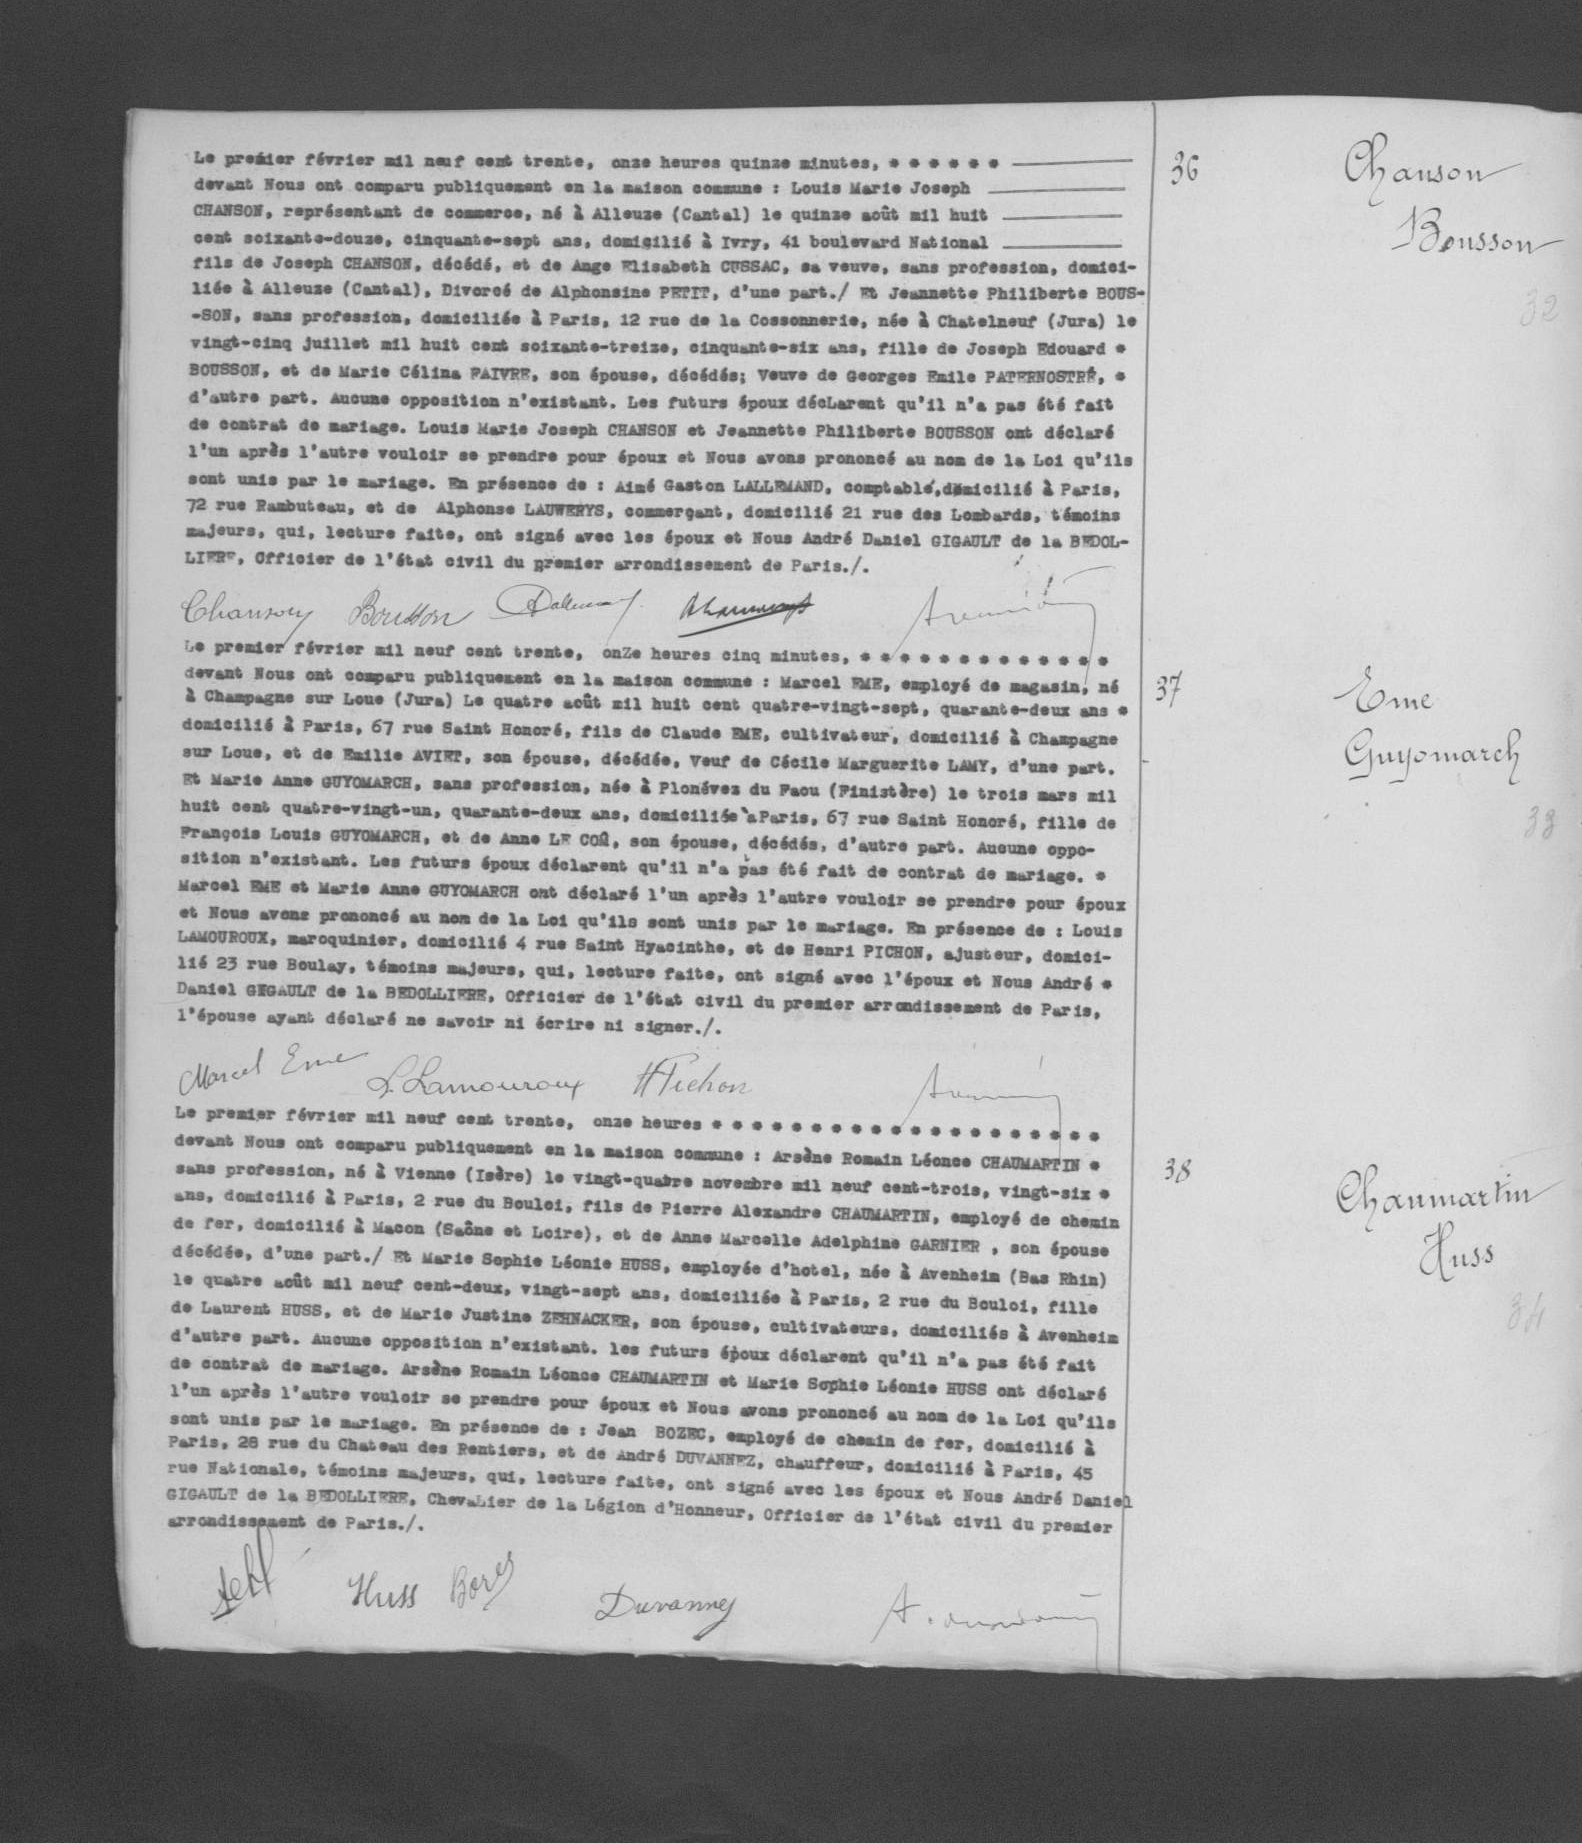Le premier février mil neuf cent trente, onze heures quinze minutes, **** -
devant Nous ont comparu publiquement en la maison commune: Louis Marie Joseph -
CHANSON, représentant de commerce, né à Alleuze (Cantal) le quinze août mil huit -
cent soixante-douze, cinquante-sept ans, domicilié à Ivry, 41 boulevard National -
fils de Joseph CHANSON, décédé, et de Ange Elisabeth CUSSAC, sa veuve, sans profession, domici-
lié à Alleuze (Cantal), Divorcé de Alphonsine PETIT, d'une part ./ Et Jeannette Philiberte BOUS-
-SON, sans profession, domiciliée à Paris, 12 rue de la Cossonnerie, née à Chatelneuf (Jura) le
vingt-cinq juillet mil huit cent soixante-treize, cinquante-six ans, fille de Joseph Edouard *
BOUSSON, et de Marie Céline FAIVRE, son épouse, décédés; Veuve de Georges Emile PATERNOSTRE, *
d'autre part. Aucune opposition n'existant. Les futurs époux déclarent qu'il n'a pas été fait
de contrat de mariage. Louis Marie Joseph CHANSON et Jeannette Philiberte BOUSSON ont déclaré
l'un après l'autre vouloir se prendre pour époux et Nous avons prononcé au nom de la Loi qu'ils
sont unis par le mariage. En présence de: Aimé Gaston LALLEMAND, comptable, domicilié à Paris,
72 rue Rambuteau, et de Alphonse LAUWERYS, commerçant, domicilié 21 rue des Lombards, témoins
majeurs, qui, lecture faite, ont signé avec les époux et Nous André Daniel GIGAULT de la BEDOL-
LIERE, Officier de l'état civil du premier arrondissement de Paris ./.
Le premier février mil neuf cent trente, onze heures cinq minutes, ****
devant Nous ont comparu publiquement en la maison commune: Marcel EME, employé de magasin, né
à Champagne sur Loue (Jura) Le quâtre août mil huit cent quatre-vingt-sept, quarante-deux ans *
domicilié à Paris, 67 rue Saint Honoré, fils de Claude EME, cultivateur, domicilié à Champagne
sur Loue, et de Emilie AVIRT, son épouse, décédée. Veuf de Cécile Marguerite LAMY, d'une part.
Et Marie Anne GUYOMARCH, sans profession, née à Polneves du Faou (Finistère) le trois mars mil
huit cent quatre-vingt-un, quarante-deux ans, domiciliée à Paris, 67 rue Saint Honoré, fille de
François Louis GUYOMARCH, et de Anne LE COM, son épouse, décédés, d'autre part. Aucune oppo-
sition n'existant. Les futurs époux déclarent qu'il n'a pas été fait de contrat de mariage. *
Marcel EME et Marie Anne GUYOMARCH ont déclaré l'un après l'autre vouloir se prendre pour époux
et Nous avons prononcé au nom de la Loi qu'ils sont unis par le mariage. En présence de: Louis
LAMOUROUX, maroquinier, domicilié 4 rue Saint Hyacinthe, et de Henri PICHON, ajusteur, domici-
lié 23 rue Boulay, témoins majeurs, qui, lecture faite, ont signé avec l'époux et Nous André *
Daniel GIGAULT de la BEDOLLIERE, Officier de l'état civil du premier arrondissement de Paris,
l'épouse ayant déclaré ne savoir ni écrire ni signer ./.
Le premier février mil neuf cent trente, onze heures ****
devant Nous ont comparu publiquement en la maison commune: Arsène Romain Léonce CHAUMARTIN *
sans profession, né à Vienne (Isère) le vingt-quatre novembre mil neuf cent-trois, vingt-six *
ans, domicilié à Paris, 2 rue du Bouloi, fils de Pierre Alexandre CHAUMARTIN, employé de chemin
de fer, domicilié à Macon (Saône-et-Loire), et de Anne Marcelle Adelphine GARNIER, son épouse
décédée, d'une part ./ Et Marie Sophie Léonie HUSS, employée d'hotel, née à Avenheim (Bas Rhin)
le quatre août mil neuf cent-deux, vingt-sept ans, domiciliée à Paris, 2 rue du Bouloi, fille
de Laurent HUSS, et de Marie Justine ZEHNACKER, son épouse, cultivateurs, domiciliés à Avenheim
d'autre part. Aucune opposition n'existant. les futurs époux déclarent qu'il n'a pas été fait
de contrat de mariage. Arsène Romain Léonce CHAUMARTIN et Marie Sophie Léonie HUSS ont déclaré
l'un après l'autre vouloir se prendre pour époux et Nous avons prononcé au nom de la Loi qu'ils
sont unis par le mariage. En présence de: Jean Bozec, employé de chemin de fer, domicilié à
Paris, 28 rue du Chateau des Rentiers, et de André DUVANNEZ, chauffeur, domicilié à Paris, 45
rue Nationale, témoins majeurs, qui, lecture faite, ont signé avec les époux et Nous André Daniel
GIGAULT de la BEDOLLIERE, Chevalier de la Légion d'Honneur, Officier de l'état civil du premier
arrondissement de Paris ./.
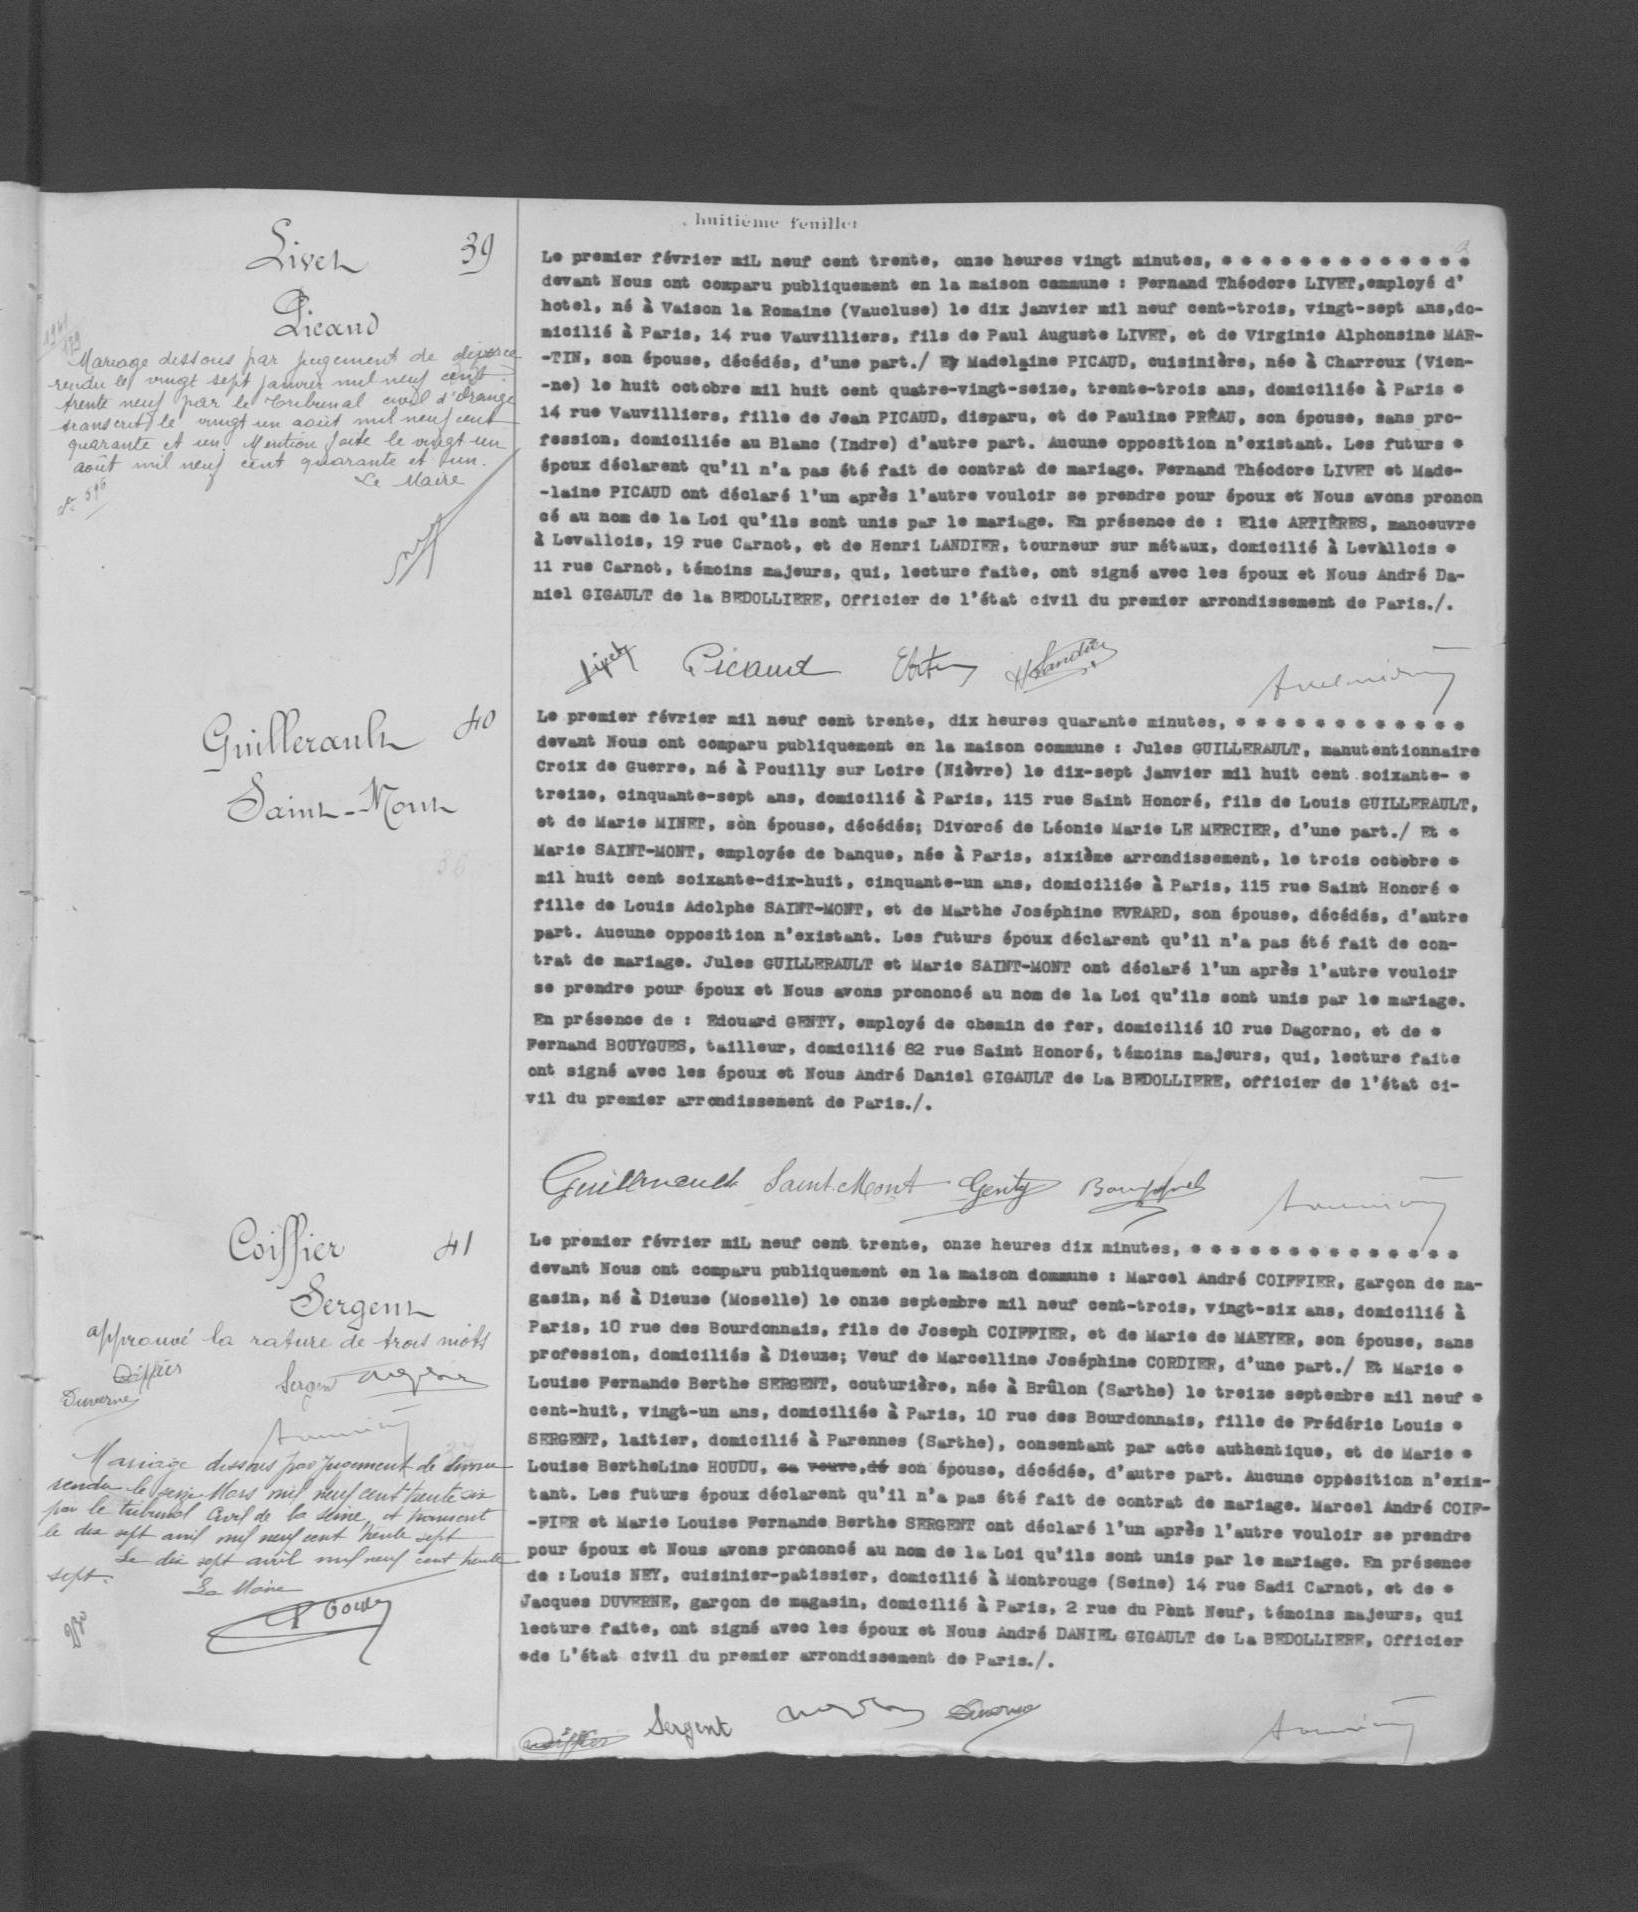Le premier février mil neuf cent trente, onze heures vingt minutes, ****
devant Nous ont comparu publiquement en la maison commune: Fernand Théodore LIVET, employé d'
hotel, né à Vaison la Romaine (Vaucluse) le dix janvier mil neuf cent-trois, vingt-sept ans, do-
micilié à Paris, 14 rue Vauvilliers, fils de Paul Auguste LIVET, et de Virginie Alphonsine MAR-
-TIN, son épouse, décédés, d'une part ./ Et Madelaine PICAUD, cuisinière, née à Charroux (Vien-
-ne) le huit octobre mil huit cent quatre-vingt-seize, trente-trois ans, domiciliée à Paris *
14 rue Vauvilliers fille de Jean PICAUD, disparu, et de Pauline PREAU, son épouse, sans pro-
fession, domiciliée au Blanc (Indre) d'autre part. Aucune opposition n'existant. Les futurs *
époux déclarent qu'il n'a pas été fait de contrat de mariage. Fernand Théodore LIVET et Made-
-laine PICAUD ont déclaré l'un après l'autre vouloir se prendre pour époux et Nous avons pronon
cé au nom de la Loi qu'ils sont unis par le mariage. En présence de: Elie ARTIERES, manoeuvre
à Levallois, 19 rue Carnot, et de Henri LANDIER, tourneur sur métaux, domicilié à Levallois *
11 rue Carnot, témoins majeurs, qui, lecture faite, ont signé avec les époux et Nous André Da-
niel GIGAULT de la BEDOLLIERE, Officier de l'état civil du premier arrondissement de Paris ./.
Le premier février mil neuf cent trente, dix heures quarant minutes, ****
devant Nous ont comparu publiquement en la maison commune: Jules GUILERAULLT, manutentionire
Croix de Guerre, né à Pouilly sur Loire (Nièvre) le dix-sept janvier mil huit cent soixante-*
treize, cinquante-sept ans, domicilié à Paris, 115 rue Saint Honoré, fils de Louis GUILLERAULT,
et de Marie MINET, son épouse, décédés; Divorcé de Léonie Marie LE MERCIER, d'une part ./ Et *
Marie SAINT-MONT, employée de banque, née à Paris, sixième arrondissement, le trois octobre *
mil huit cent soixante-dix-huit, cinquante-un ans, domiciliée à Paris, 115 rue SAint Honoré *
fille de Louis Adolphe SAINT-MONT, et de Marthe Joséphine EVRARD, son épouse, décédés, d'autre
part. Aucune opposition n'existant. Le futurs époux déclarent qu'il n'a pas été fait de cont
rat de mariage. Jules GUILLERAULT et Marie SAINT-MONT ont déclaré l'un après l'autre vouloir
se prendre pour époux et Nous avons prononcé au nom de la Loi qu'ils sont unis par le mariage.
En présence de: Edouard GENTY, employé de chemin de fer, domicilié 10 rue DAGORNO, et de *
Fernand BOUYGUES, tailleur, domicilié 82 rue Saint Honoré, témoins majeurs, qui, lecture faite
ont signé avec les époux et Nous André Daniel GIGAULT de la BEDOLLIERE, officier de l'état ci-
vil du premier arrondissement de Paris ./.
Le premier février mil neuf cent trente, onze heures dix minutes, ****
devant Nous ont comparu publiquement en la maison commune: Marcel André COIFFIER, garçon de ma-
gasin, né à Dieuse (Moselle) le onze septembre mil neuf cent-trois, vingt-six ans, domicilié à
Paris, 10 rue des Bourdonnais, fils de Joesph COIFFIER, et de Marie de MAEYER, son épouse, sans
profession, domiciliée à Dieuse; Veuf de Marcelline Joséphine CORDIER, d'une part ./ Et Marie *
Louise Fernande Berthe SERGENT, couturière, née à Brûlon (Sarthe), le treize septembre mil neuf *
cent-huit, vingt-un ans, domiciliée à Paris, 10 rue des Bourdonnais, fille de Frédéric Louis *
SERGENT, laitier, domicilié à Parennes (Sarthe), consentant par acte authentique, et de Marie *
Louise Bertheline HOUDU, son épouse, décédée, d'autre part. Aucune opposition n'exis-
tant. Les futurs époux déclarent qu'il n'pas été fait de contrat de mariage. Marcel André COIF-
-FIER et Marie Louise Fernaned Berthe SERGENT ont déclaré l'un après l'autre vouloir se prendre
pour époux et Nous avons prononcé au nom de la Loi qu'ils sont unis par le mariage. En présence
de: Lois NEY, cuisinier-patissier, domicilié à Montrouge (Seine) 14 rue Sadi Carnot, et de *
Jacques DUVERNE, garçon de magasin, domicilié à Paris, 2 rue du Pont Neuf, témoins majeurs, qui
lecture faite, ont signé avec les époux et Nous André DANIEL Gigault de la BEDOLLIERE, Officier
de l'état civil du premier arrondissement de Paris ./.